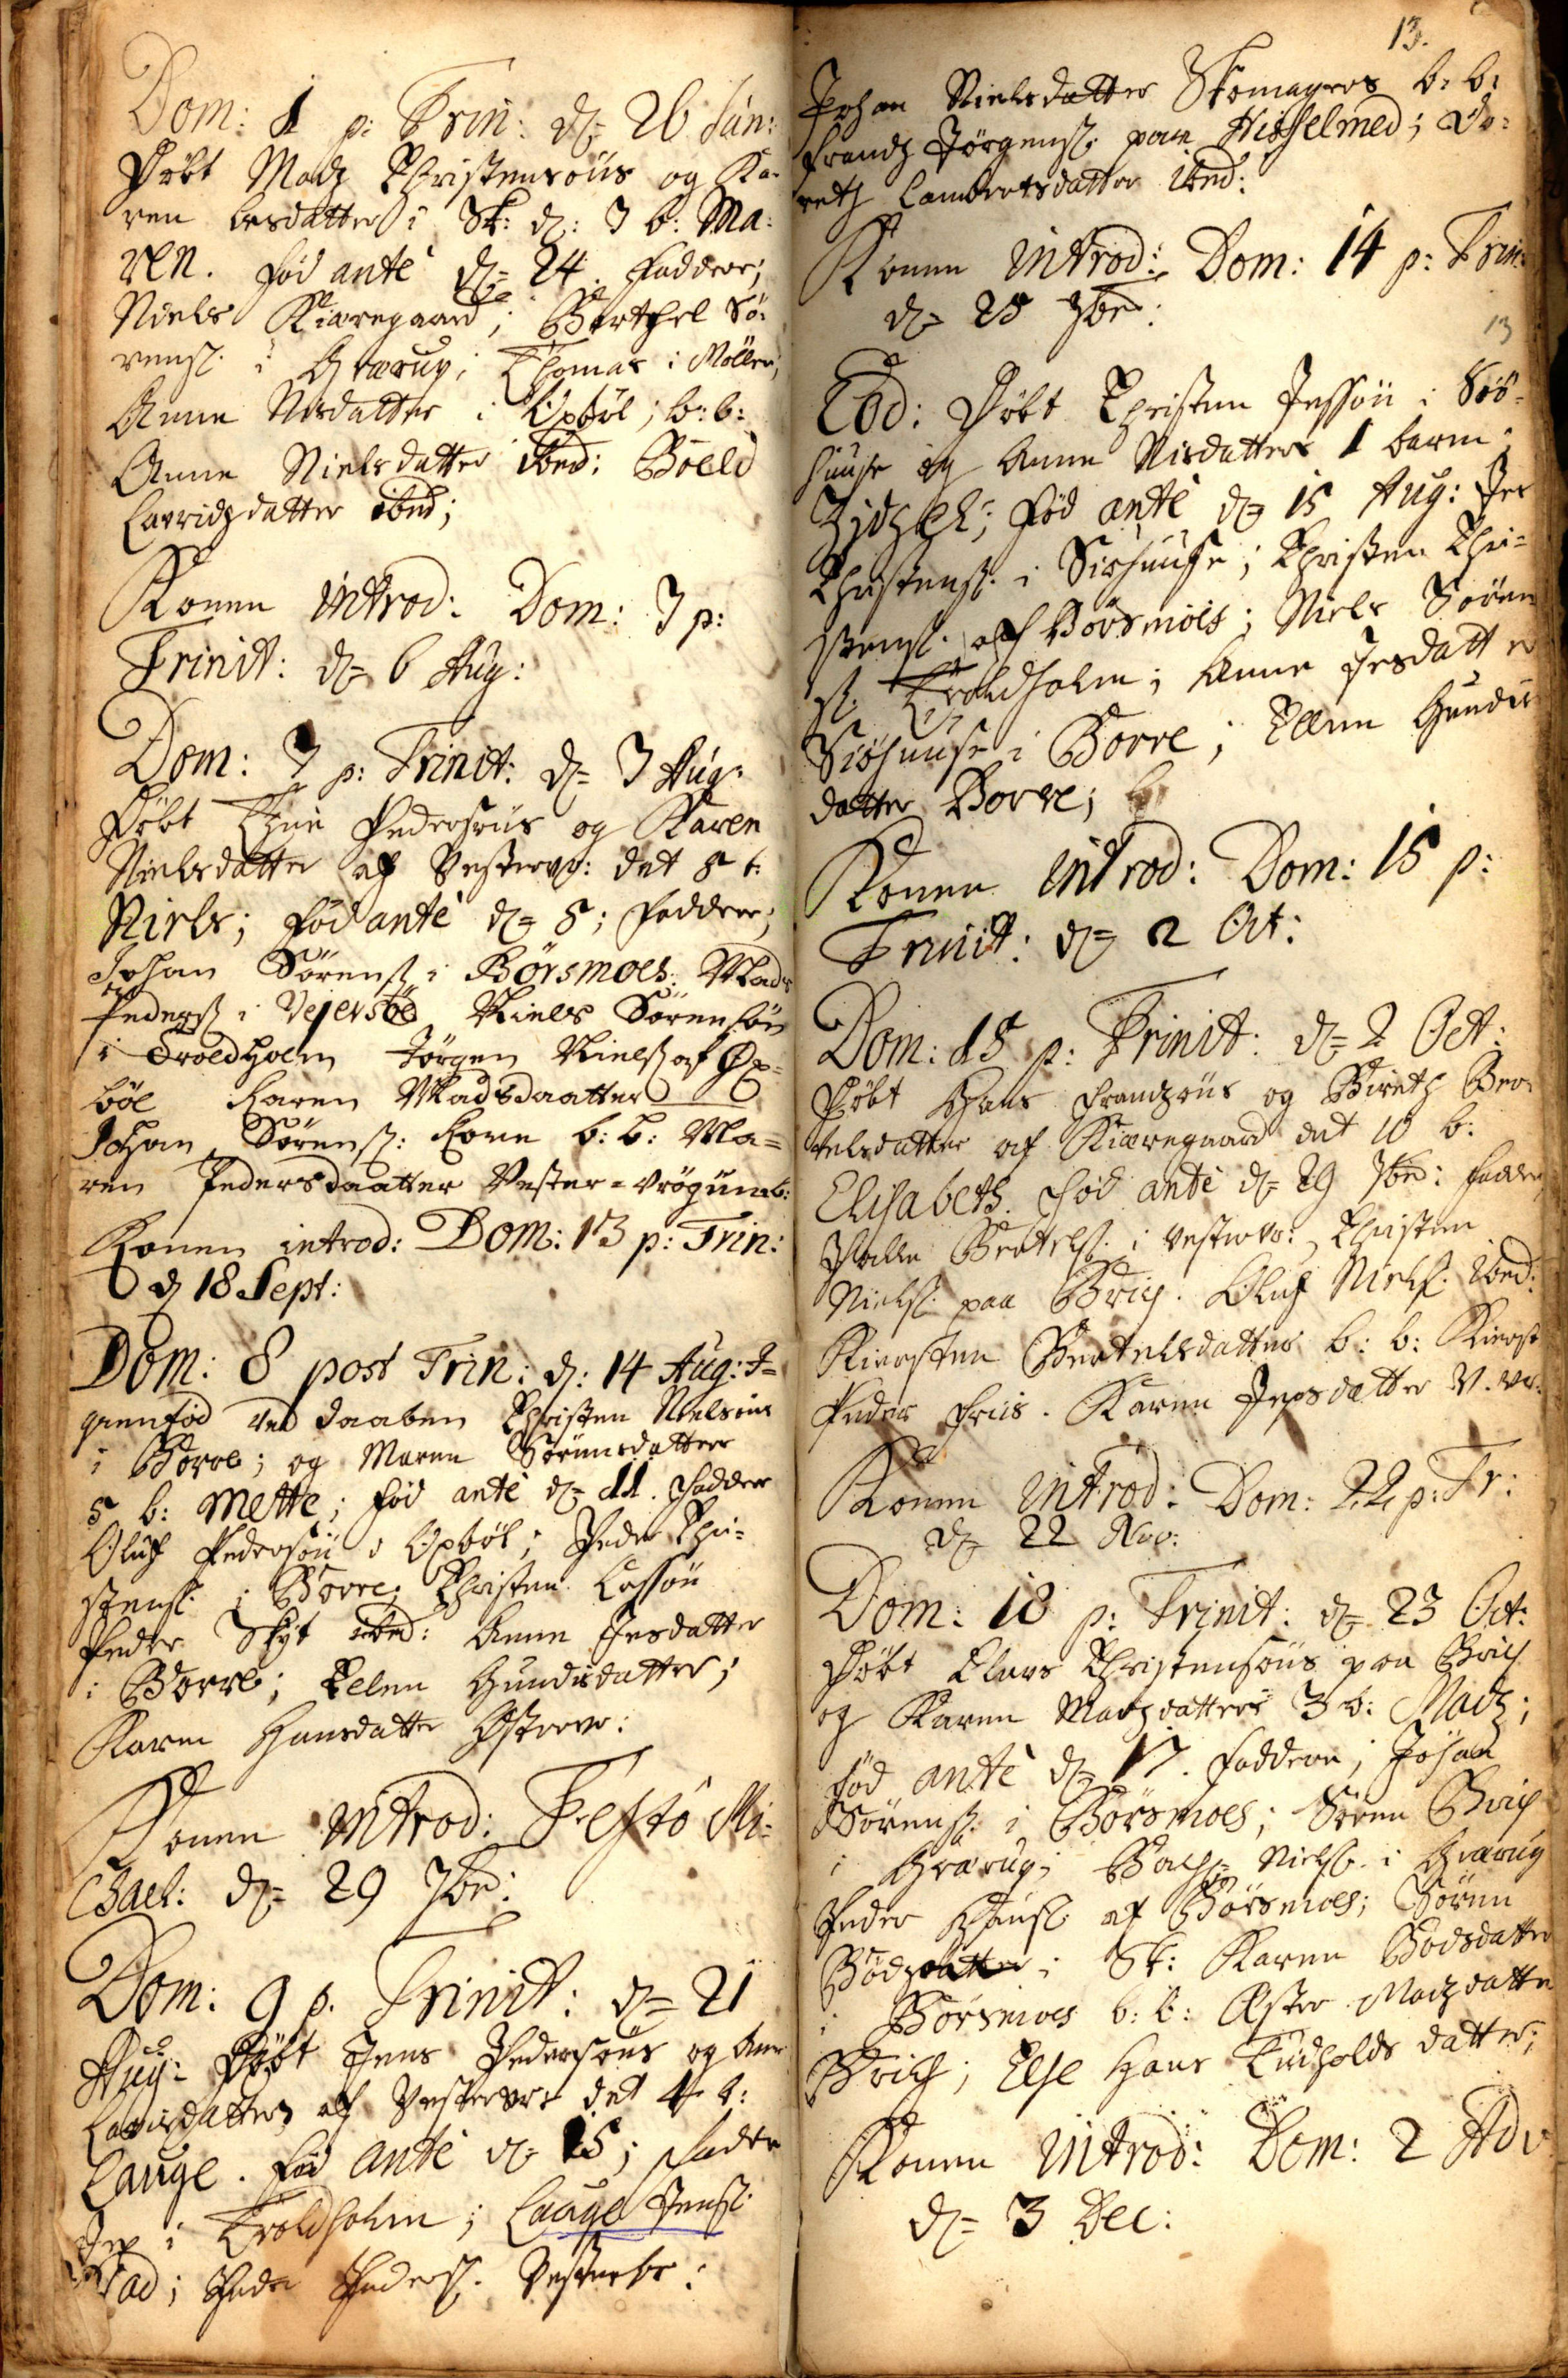Dom: 1 p:. Trin: d 26 Jun:
Døbt Madz Christensøns og Ka¬
ren Lasdatter i Sk: d: 3 b. Ma¬
ren. fød ante d= 24. Faddere;
Niels Kiæregaard; Berthel Sø¬
rens i Grærup; Thomas i Møllen,
Anne Nisdatter i Oxbøl b: b:
Anne Nielsdatter ibm: Boeld
Lavridzdatter ibm;
Konen introd: Dom: 7 p:
Trinit: d= 6 Aug:
Dom: 7 p: Trinit: d= 7 Aug:
Døbt Thue Pedersøns og Karen
Nielsdatter af Vestervr: det 5 b:
Niels; fød ante d= 5; Faddere;
Johan Sørens i Børsmoes: Mads
Peders i Vejersøe, Niels Sørensøn
i Troldholm Jørgen Niels af Ox¬
bøl Karen Madsdaatter
Johan Sørens: Kone b: B: Ma¬
ren Pedersdaatter Vester=Vrøgum#:
Konen introd: Dom: 13 p: Trin:
d 18 Sept:
Dom: 8 post Trin: d:14 Aug. I¬
gienfød ved Daaben Christen Nielsøns
i Borre; og Maren Sørensdatters
5 b: Mette; fød ante d= 11. Faddere
Oluf Pedersøn i Oxbøl; Peder Chri¬
stens i Borre; Christen Lassøn
Peder Skyt ibm: Anne Jesdatter
i Borre; Ellen Gundisdatter;
Karen Hansdatter Østervr:
Konen introd: Festo Mi¬
chael: d= 29 7br:
Dom: 9 p. Trinit: d= 21
Aug: Døbt Jens Pedersøns og Anne
Lavisdatters af Vestervr: det 4 b:
Lauge. fød ante d= 15; Faddere
Jep i Troldholm; Lauge Jens
Vad; Peder Peders Vestervr:
13
13
Johan Nielsdatter Skomagers b: b:
Frandz Jørgens paa Hisselmed; Do¬
reth Lambertsdatter ibm.
Konen introd: Dom: 14 p: Trin:
d= 25 7br:
Eod: Døbt Christen Jessøn i Siø¬
huuse og Anne Nisdatters 1 Barn;
Zidzel; fød ante d= 15 Aug: Jes
Christens i Siohuuse; Christen Chri¬
stens af Børsmoes; Niels Søren¬
s Troldholm; Anne Jesdatter
Siøhuuse i Borre; Ellen Gundis
datter Borre; b
Konen introd: Dom: 15 p:
Trinit: d= 2 Oct:
Dom: 15 p: Trinit: d= 2 Oct:
Døbt Hans Frandzøns og Bireth Ber¬
telsdatter af Kiæregaard det 10 b:
Elisabeth. fød ante d= 29 7br.: Faddere
Palle Bertels i Vestervr: Christen
Nielsen paa Brich,.Oluf Niels ibm,
Kiersten Bertelsdatter b: b: Kierst
Peder Frii. Karen Jepsdatter V.vr.
Konen introd: Dom: 22 p: Tr.:
d= 22 Nov:
Dom:18 p: Trinit: d= 23 Oct:
Døbt Claus Christensøns paa Brich
og Karen Madzdatters 3 b: Madz;
fød ante d= 17. Faddere; Johan
Sørens i Børsmoes; Søren Brich
i Grærup; Bach= Niels i Grærup,
Peder Hans af Børsmoes; Søren
Bodz## i Sk: Karen Bodsdatter
i Børsmoes b: b: Æster Madzdatter;
Brich; Else Hans Tudholds Datter.
Konen introd: Dom: 2 Adv
d= 3 Dec:
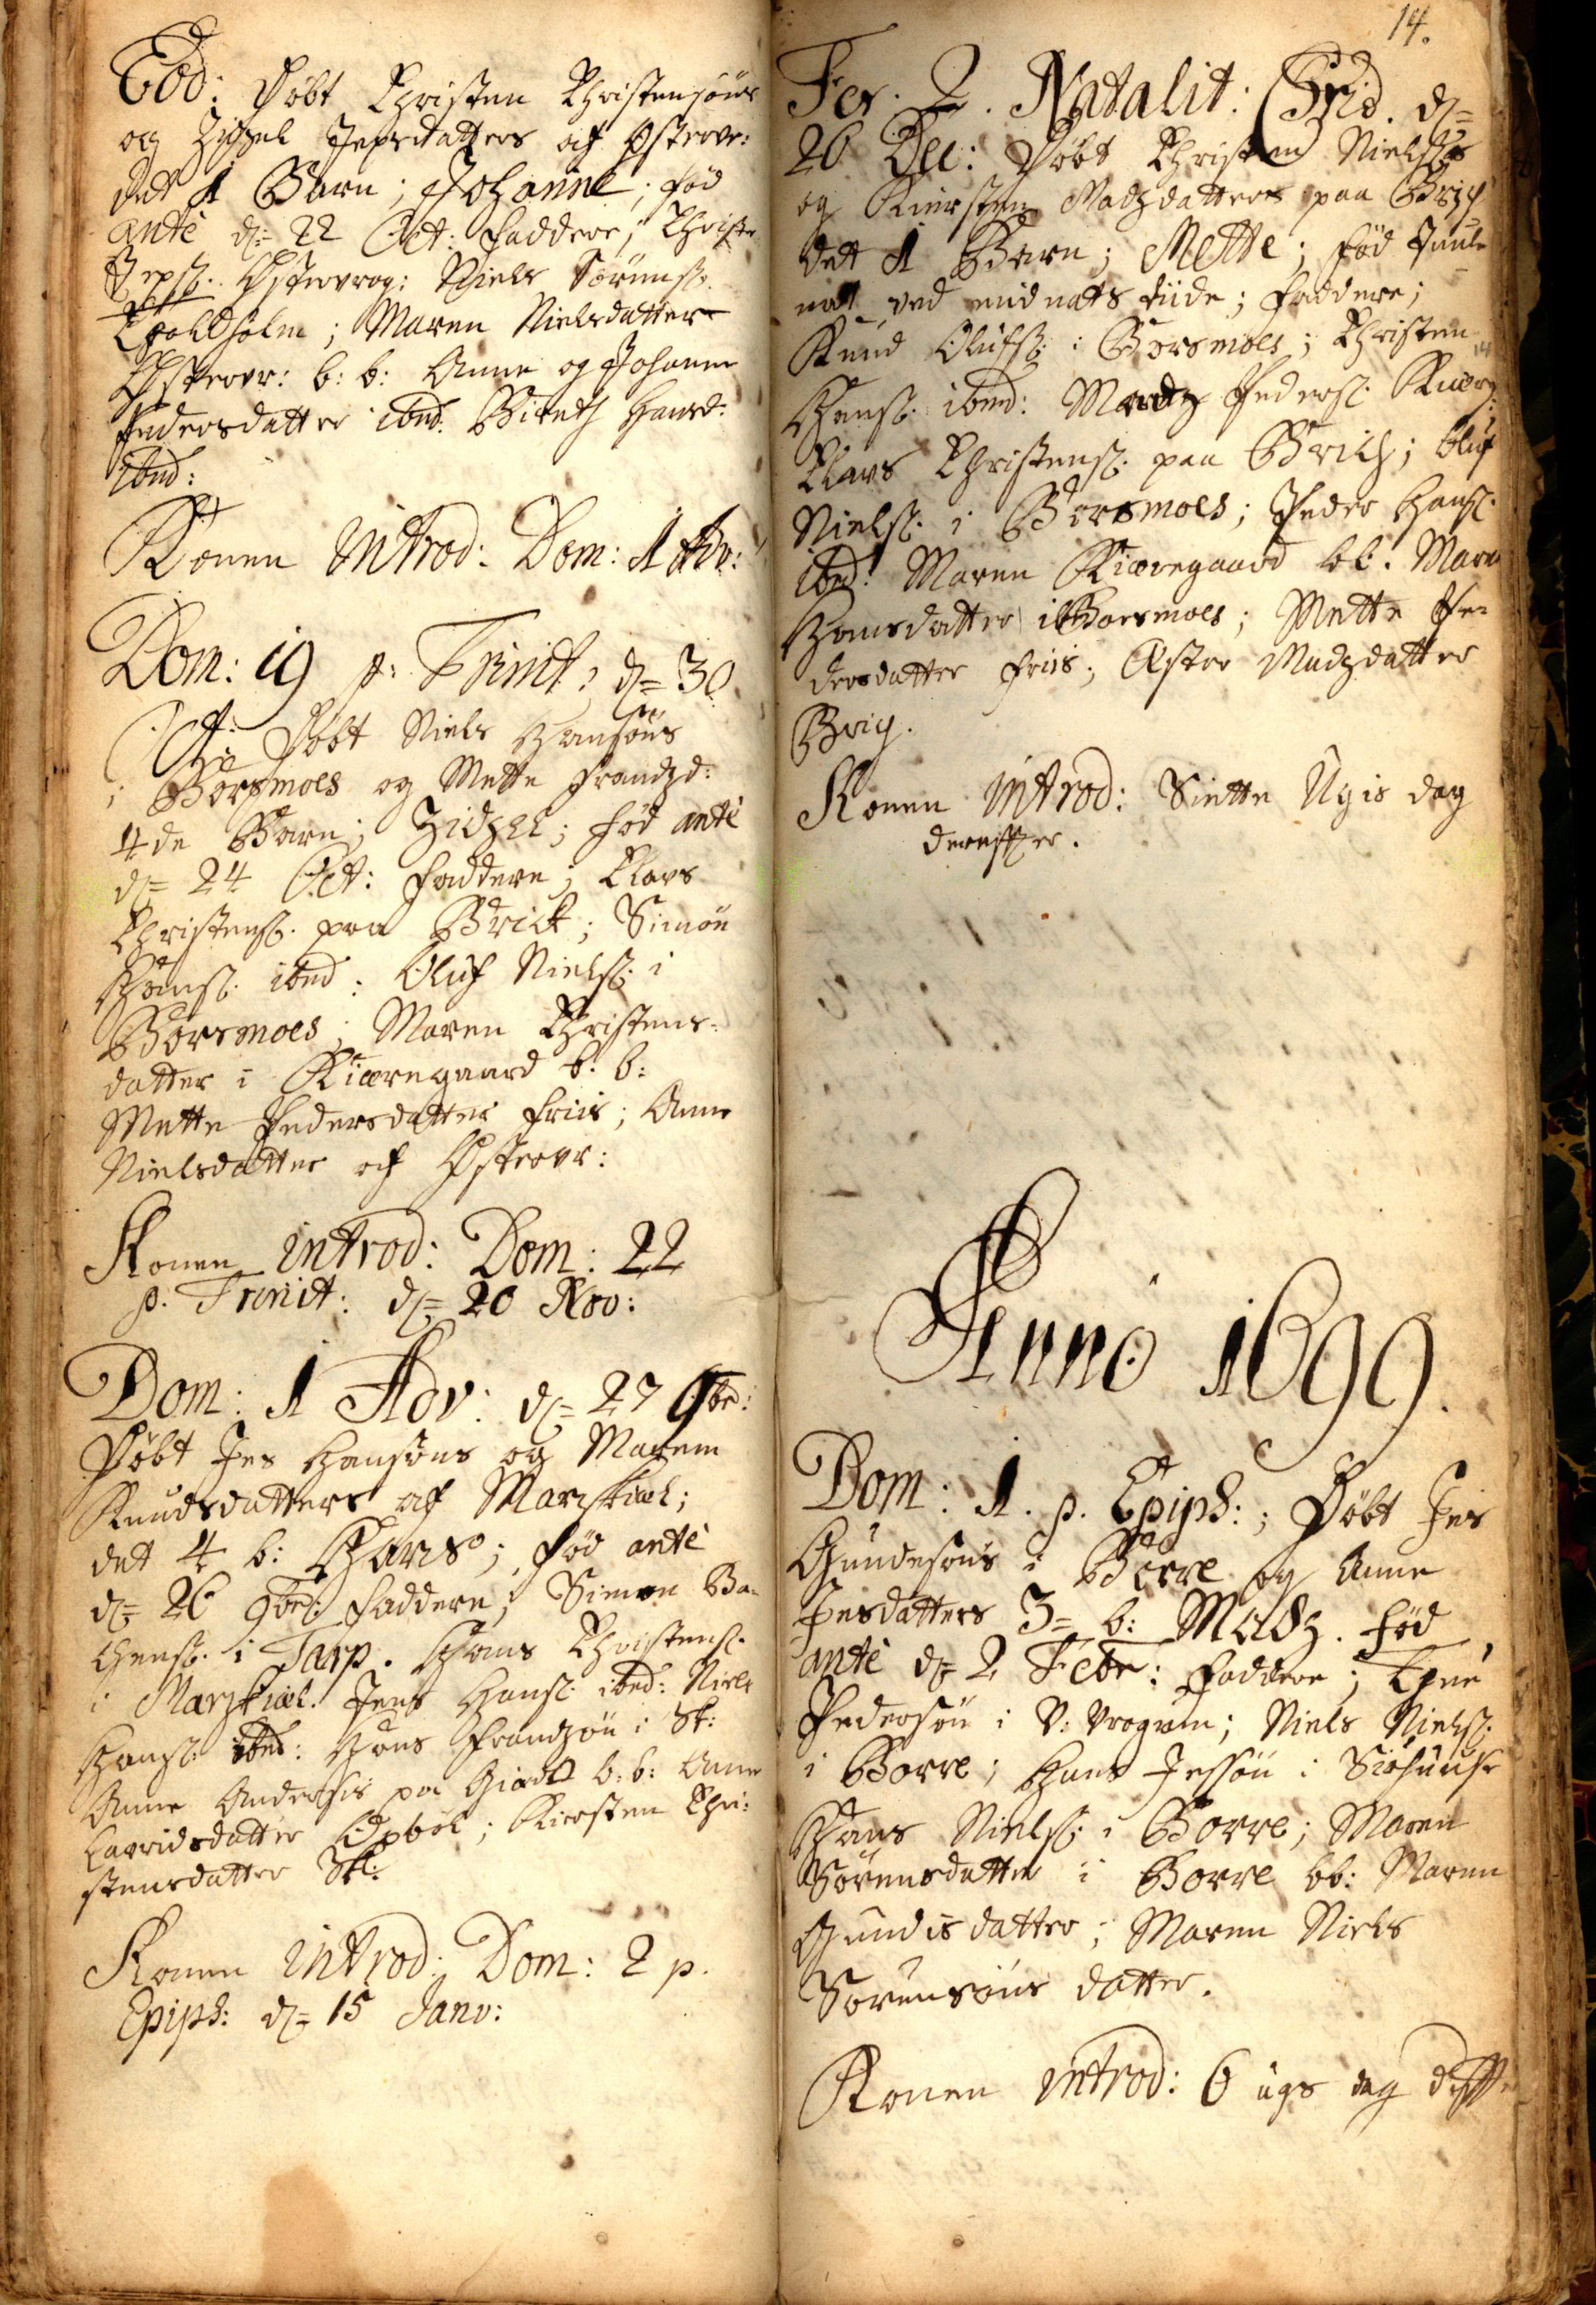Eod: Døbt Christen Christensøns
og Zidzel Jepsdatters af Østervr:
det 1 Barn; Johanne; fød
ante d= 22 Oct: Faddere; Christen
Jeps i Østervog: Niels Sørens
Troldholm; Maren Nielsdatter
Østervr: b: b: Anne og Johanne
Pedersdatter ibm: Bireth Hansd:
ibm:
Konen introd: Dom: 1 Adv:
Dom: 19 p: Trinit: d= .30
Oct: Døbt Niels Hansøns
i Børsmoes og Mette Frandzd:
4de Barn; Zidzel; fød ante
d= 24 Oct: Faddere; Clavs
Christens paa Brick; Simon
Hans. ibm: Oluf Niels i
Børsmoes; Maren Christens¬
datter i Kiæregaard b. B:
Mette Pedersdatter Friis; Anne
Nielsdatter af Østervr:
Konen introd: Dom: 22
p. Trinit: d= 20 Nov:
Dom: 1 Adv. d= .27 9br:
Døbt Jes Hansøns og Maren
Knudsdatters af Marskiæl;
det 4 B: Hans; fød ante
d= 26 9br: Faddere; Simon Ba¬
chens i Tarp. Hans Christens.
i Marskiæl. Jens Hansen ibm: Niels
Hansen ibm: Hans Frandzøn i Sk:
Anne Andersis paa Giedb. b: b: Anne
Lavridsdatter Oxbøl; Kiersten Chri¬
stensdatter Sk:
Konen introd: Dom: 2 p.
Epiph: d= 15 Janv:
14
14
Fes. 2. Natalit: Chrio. d=
26 Dec: Døbt Christen Niels ##
og Kiersten Madzdatters paa Brich
det 1 Barn; Mette; fød Juule
nat ved midnatstiide; Faddere;
Knud Olufs: i Børsmoes; Christen
Hans ibm: Madz Peders Kiærg:
Claus Christens paa Brich; Oluf
Niels i Børsmoes; Peder Hansen
ibid, Maren Kiæregaard bb. Maren
Hansdatter i Borsmoes; Mette Pe¬
dersdatter Friis; Æster Madzdatter
Brich.
Konen introd: Siette Ugis dag
derefter.
Anno 1699
Dom:1.p. Epiph: ; Døbt Jes
Gundesøns i Borre og Anne
Jesdatters 3= B: Madz, fød
ante d= 2 Febr: Faddere:; Thue
Pedersøn i V.Vrogum; Niels Niels.
i Borre; Hans Jessen i Siøhuuse,
Hans Niels i Borre; Maren
Sørensdatter i Borre bb: Maren
Gundisdatter; Maren Niels
Sørensøns Datter.
Konen introd: 6 ugs dag derefter